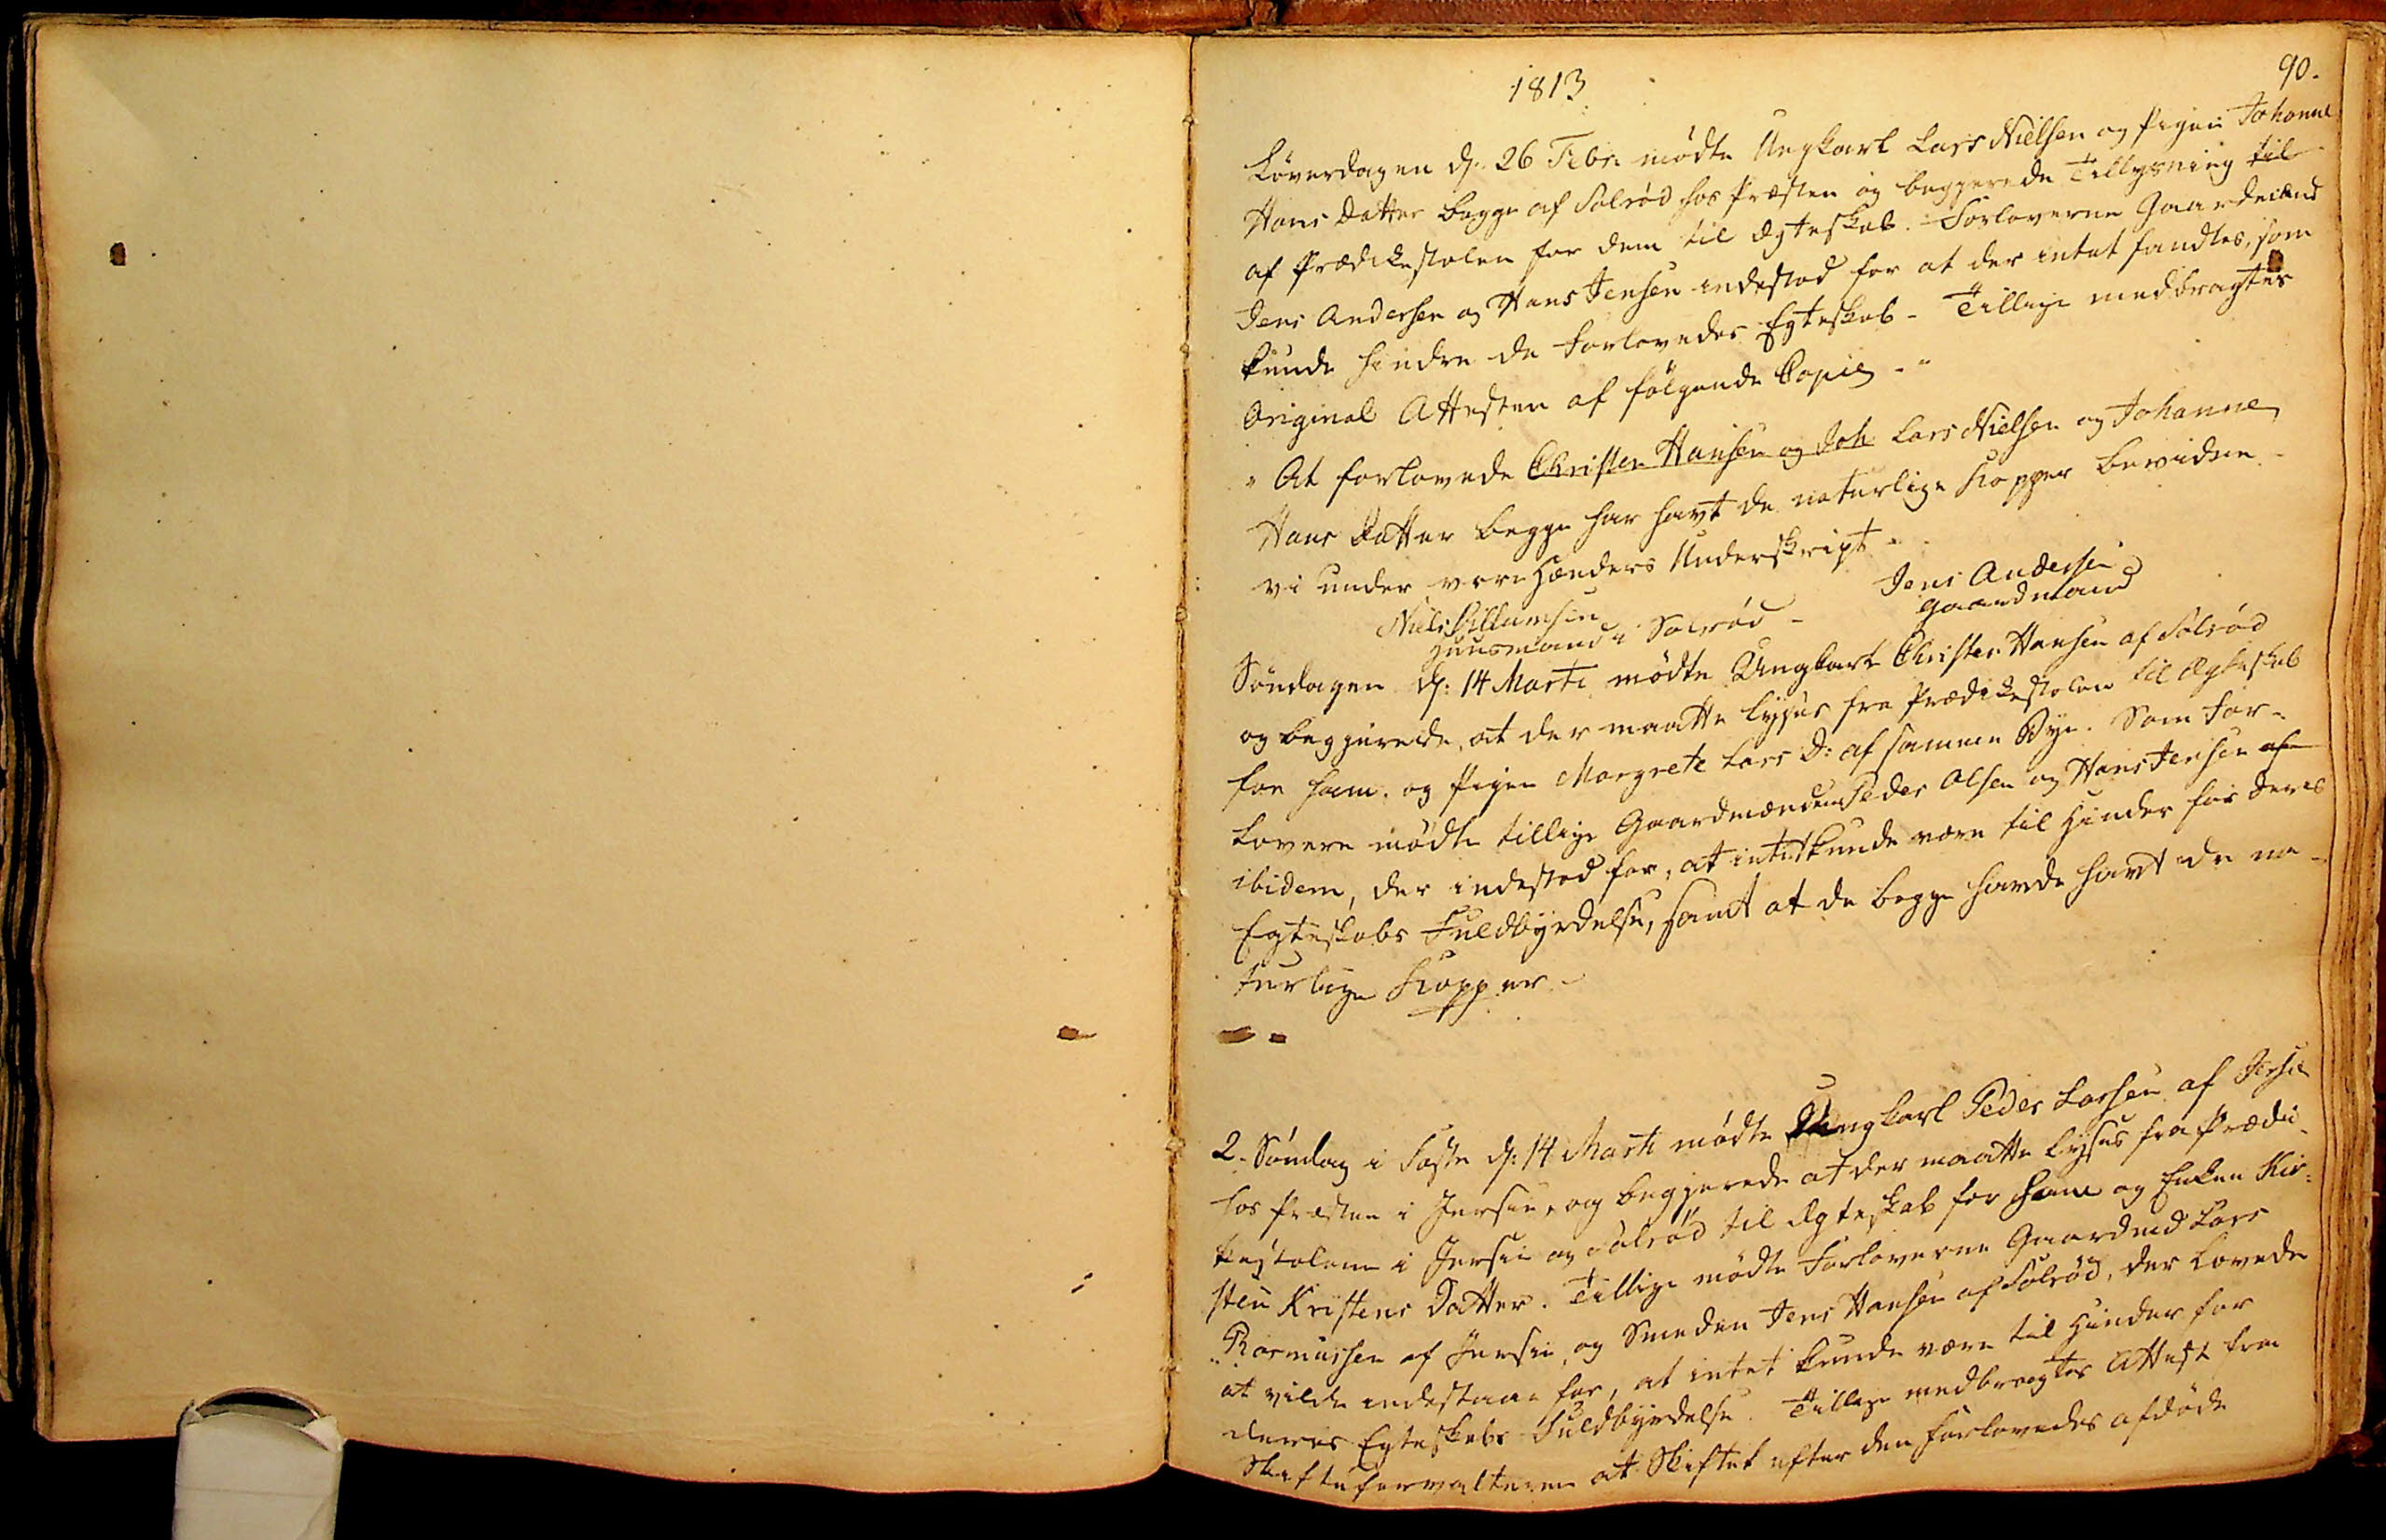90.
1813
Løverdagen d. 26 Febr: mødte Ungkarl Lars Nielsen og Pigen Johanne
Hans Datter begge af Solrød hos Præsten og begjerede Tillysning til
af Prædikestolen for dem til Ægteskab. Forloverne Gaardmænd
Jens Andersen og Hans Jensen indestod for at der intet fandtes, som
kunde hindre de forlovedes Egteskab. Tillige medbragtes
Original Attesten af følgende Copie.
,, At forlovede Christen Hansen og Joh Lars Nielsen og Johanne
Hans Datter begge har havt de naturlige Kopper bevidner
vi under vore Hænders Underskrivt.
Niels Villumsen
Jens Andersen
Huusmand i Solrød
Gaardmand
Søndag d: 14 Marts mødte Ungkarl Christen Hansen af Solrød
og begjerede, at der maatte lyses fra Prædikestolen til Ægteskab
for ham og Pigen Margrete Lars D: af samme Bye. Som For¬
lovere mødte tillige Gaardmændene Peder Olsen og Hans Jensen af
ibidem, der indestod for, at intet kunde være til Hinder for deres
Egteskabs Fuldbyrdelse, samt at de begge havde havt de na¬
turlige Kopper
2. Søndag i Faste d: 14 Marts mødte Ungkarl Peder Larsen af Jersie
hos Præsten i Jersie, og begjerede at der maatte lyses fra Prædi
kestolen i Jersie og Solrød til Ægteskab for ham og Enken Kir¬
sten Kristens Datter. Tillige mødte Forloverne Gaardmd Lars
Rasmussen af Jersie, og Smeden Jens Hansen af Solrød, der lovede
at vilde indestaae for, at intet kunde være til Hinder for
deres Egteskabs Fuldbyrdelse. Tillige medbragtes Attest fra
Skifteforvalteren at Skiftet efter den Forlovedes afdøde
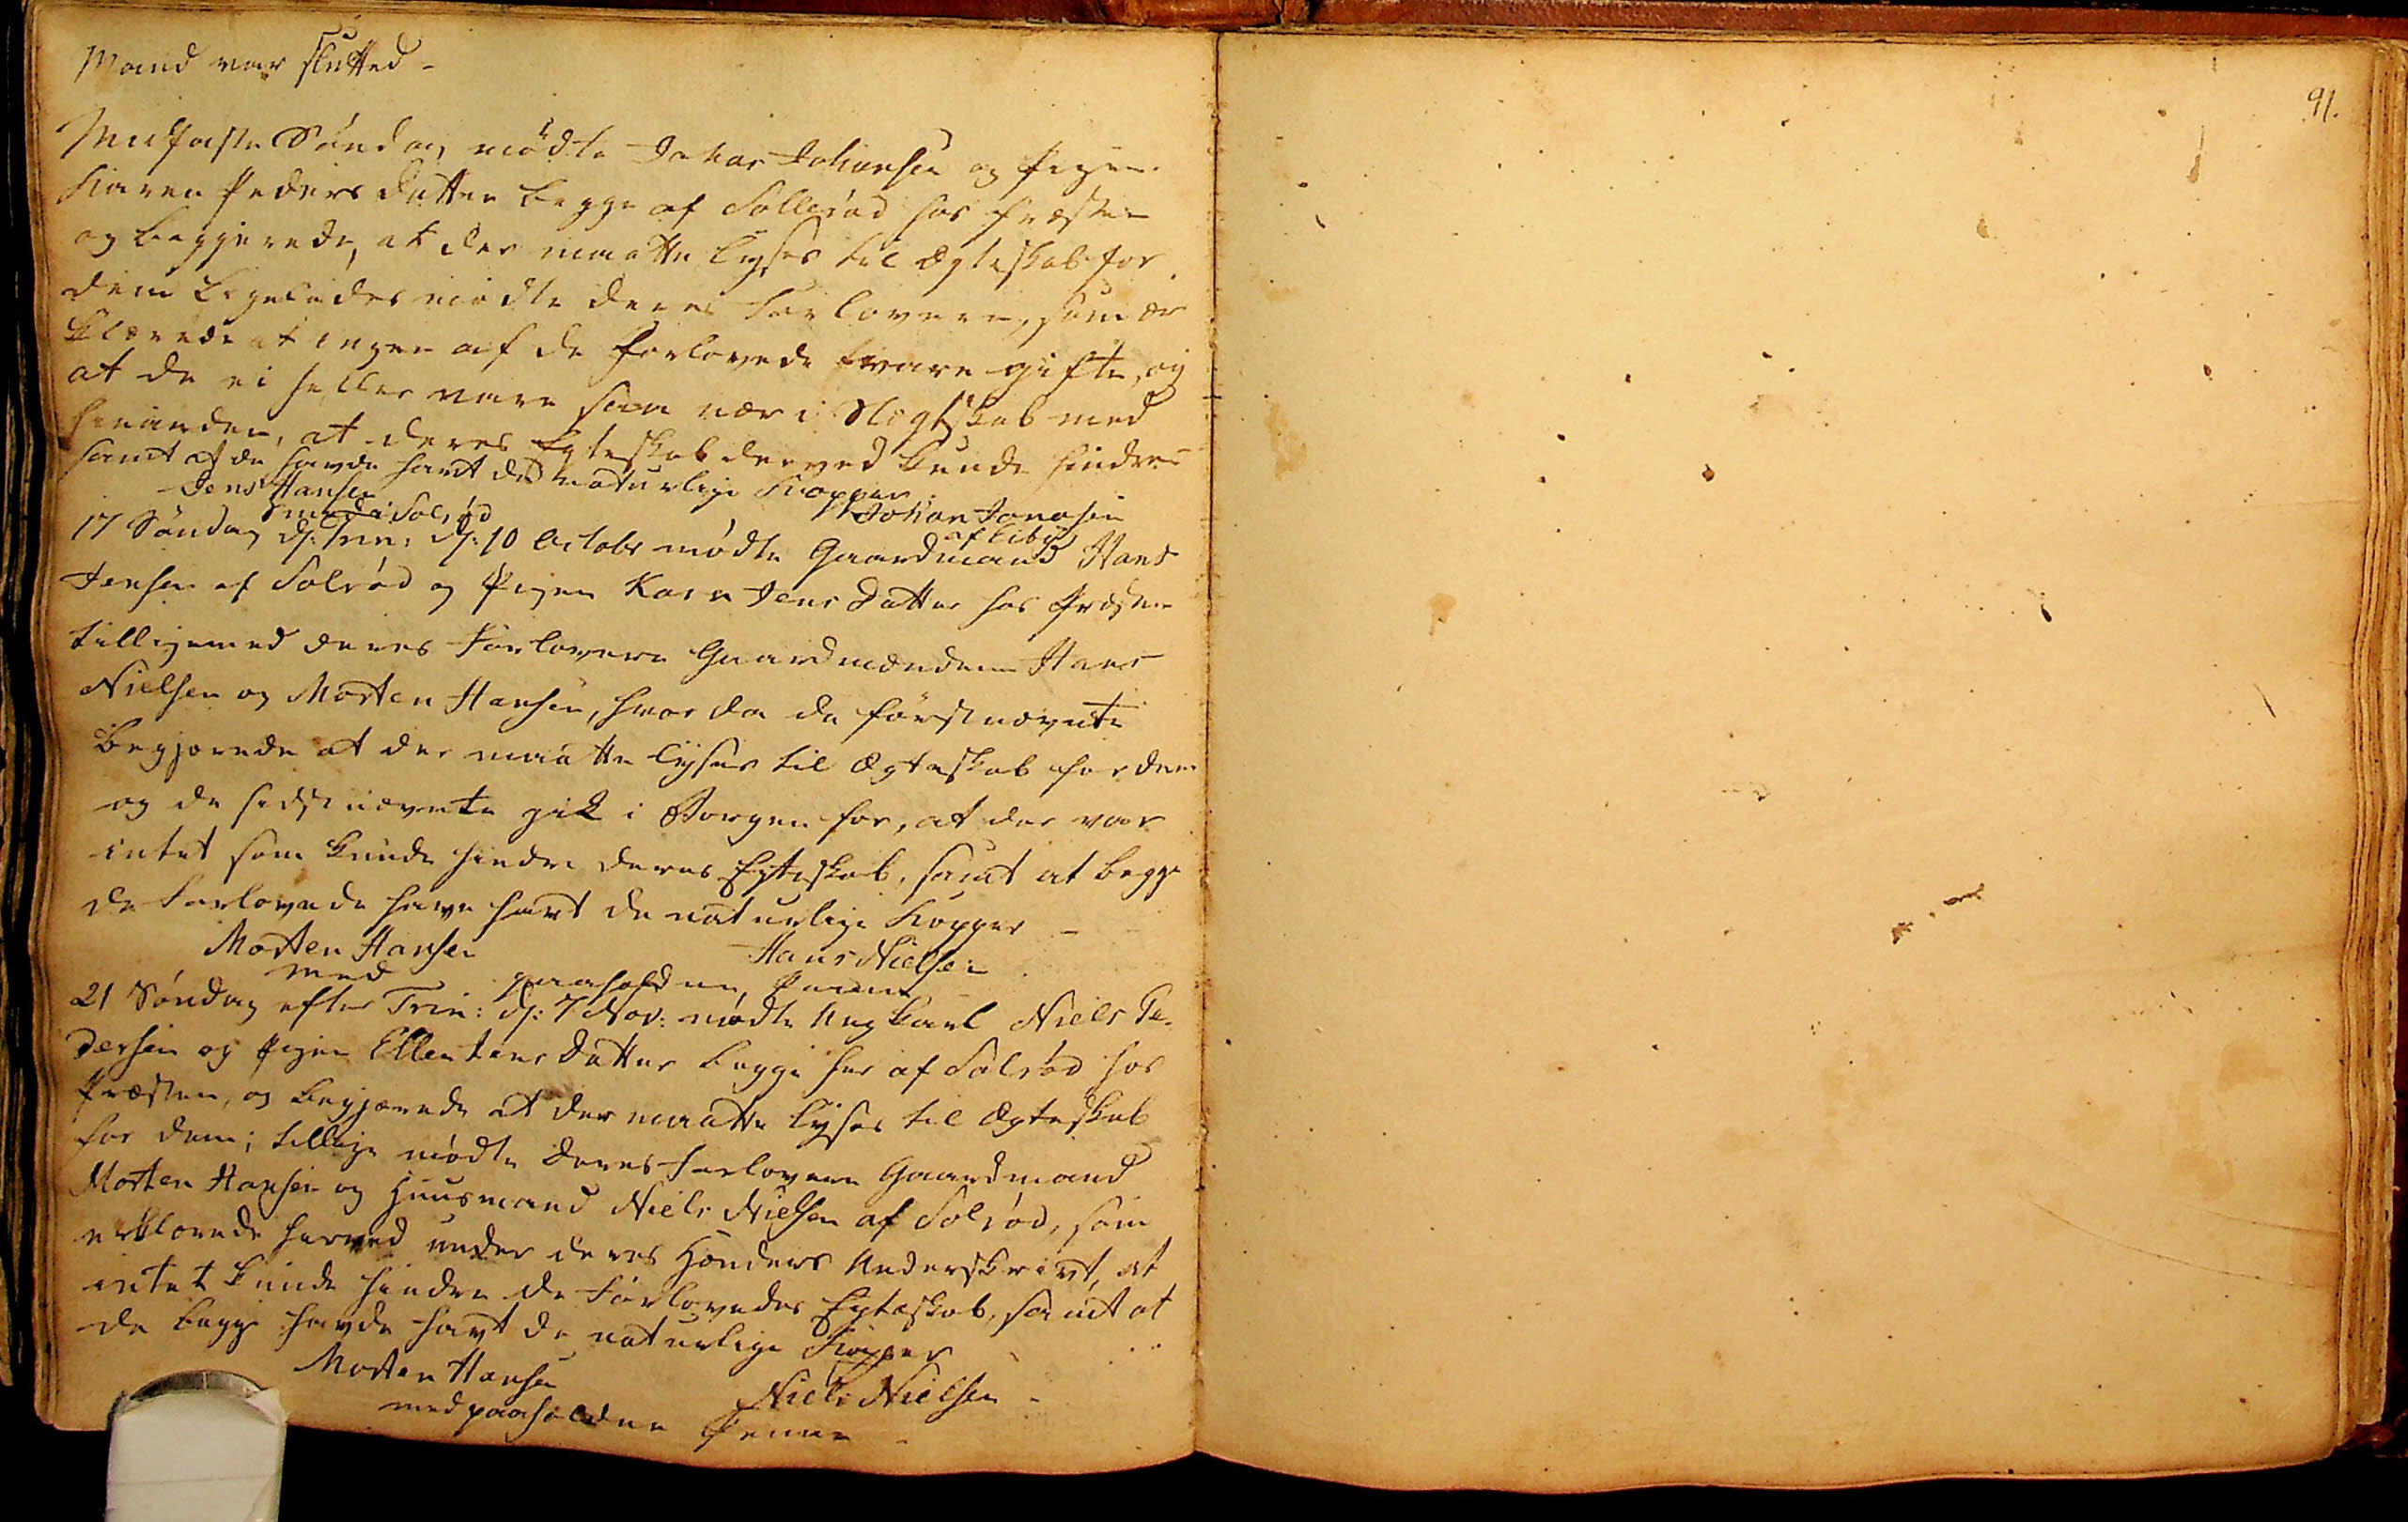Mand var slutted.
Midfaste Søndag mødte Jonas Johansen og Pigen
Karen Peders Datter begge af Sollerød hos Præsten
og begjerede, at der maatte lyses til Ægteskab for
dem Ligeledes mødte Deres Forlovere, som er
klærede at ingen af de Forlovede vare gifte, og
at de ei heller vare som nær i Slægtskab med
hinanden, at deres Egteskab derved kunde hindres
samt at de havde havt de naturlige Kopper;
Jens Hansen
Johan Jonasen
Smed i Solrød
af Eiby
17 Søndag d: Trin: d: 10 Octobr mødte Gaardmand Hans
Jensen af Solrød og Pigen Karn Jens Datter hos Præsten
tilligemed deres Forlovere Gaardmændene Hans
Nielsen og Morten Hansen, hvor da de førstnævnte
begjærede at der maatte lyses til Ægteskab for dem
og de sidstnævnte gik i Borgen, at der var
intet som kunde hindre deres Egteskab, samt at begge
de Forlovede havde havt de naturlige Kopper
Morten Hansen
Hans Nielsen
med
paaholdne Penne
21 Søndag efter Trin: d: 7 Nov: mødte Ungkarl Niels Pe¬
dersen og Pigen Ellen Jens Datter begge her af Solrød hos
Præsten, og begjærede at der maatte lyses til Ægteskab
for dem; tillige mødte deres Forlovere Gaardmand
Morten Hansen og Huusmand Niels Nielsen af Solrød, som
erklærede herved under deres Hænders Underskrivt, at
intet kunde hindre de Forlovedes Egteskab, samt at
de begge havde havt de naturlige Kopper
Morten Hansen
Niels Nielsen
med paaholdne Penne